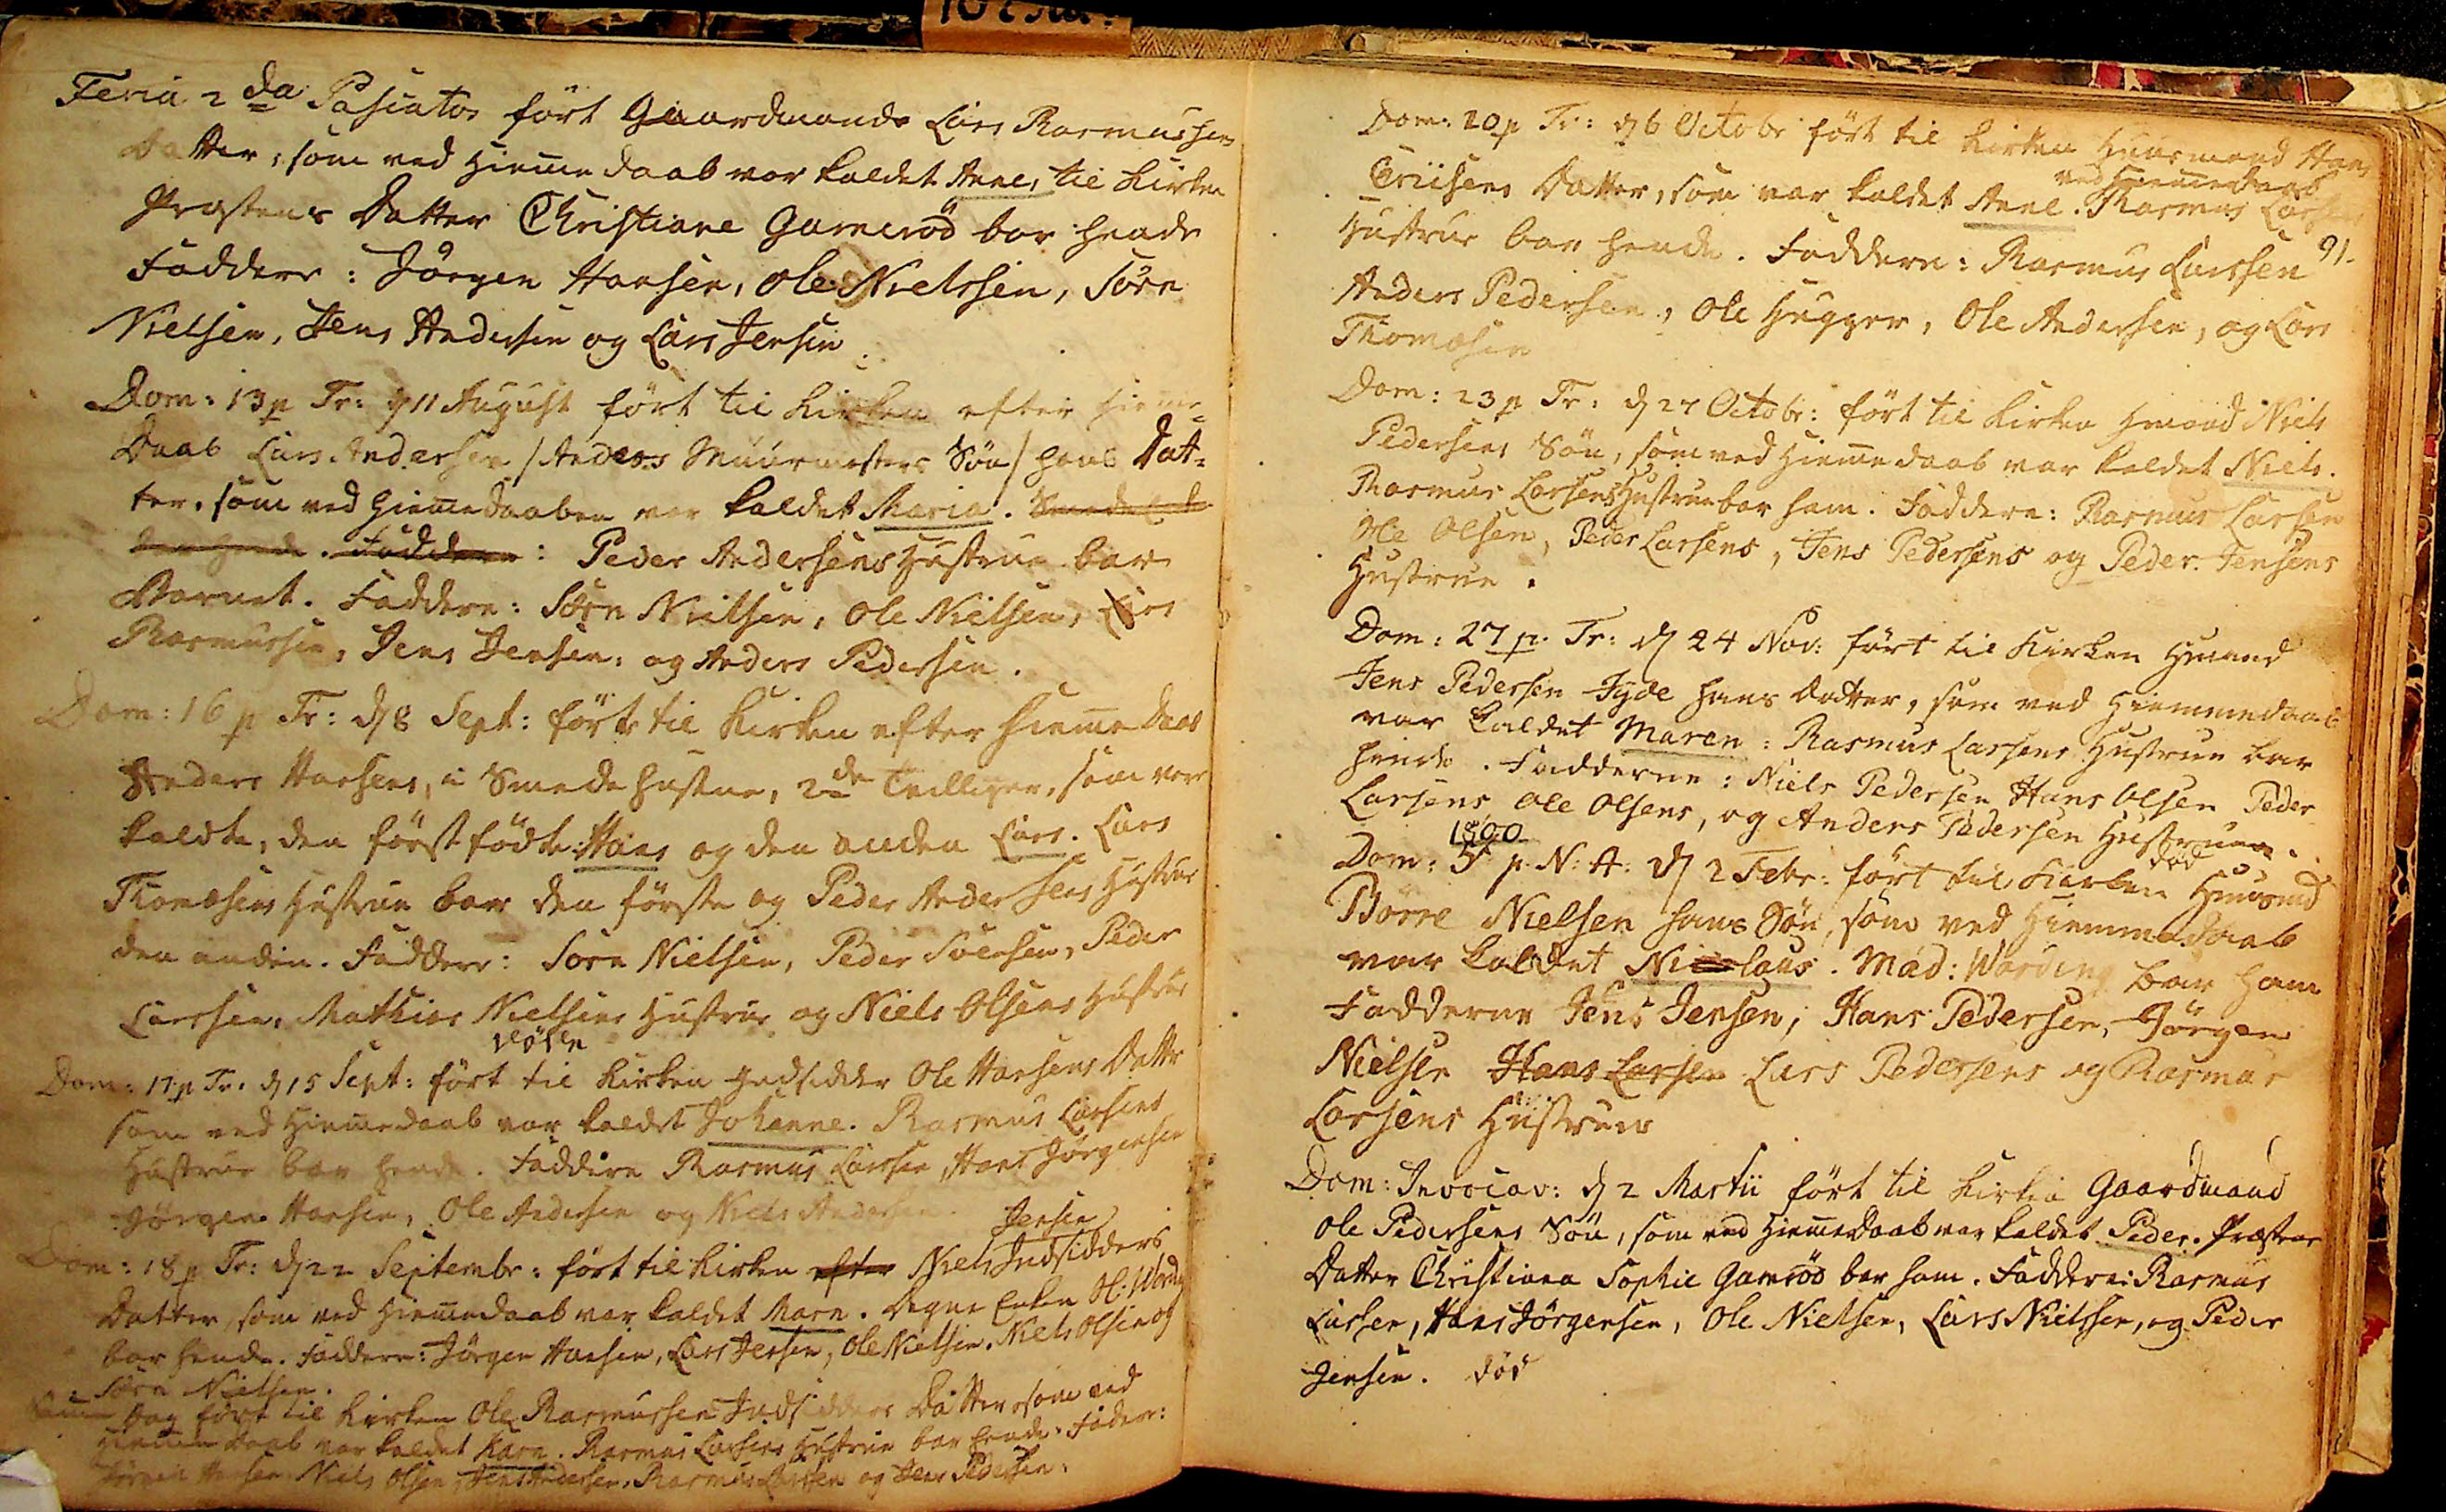Feria 2da Pascatus ført Gaardmand Lars Rasmussens
Datter, som ved Hiemmedaab var kaldet Anne, til Kirken
Præstens Datter Christiane Gamerød bar hende
Faddere: Jørgen Hansen, Ole Nielssen, Sørn
Nielsen, Jens Andersen og Lars Jensen.
Dom: 13 p Tr: d 11 August ført til Kirken efter Hiemme¬
Daab Lars Andersen / Anders Muurmesters Søn / hans Dat¬
ter, som ved Hiemmedaaben var kaldet Maria. Smede Enken
bar hende. Faddere: Peder Andersens Hustrue bar
Barnet. Faddere: Sørn Nielsen. Ole Nielsen, Lars
Rasmussen, Jens Jensen, og Anders Pedersen.
Dom: 16 p Tr: d 8 Sept: ført til Kirken efter Hiemme Daab
Anders Hansens, i Smedehusene, 2de Tvillinger, som vare
kaldte, den først fødte Hans og den anden Lars. Lars
Thomæsens Hustrue bar den første og Peder Andersens Hustrue
den anden. Faddere: Sørn Nielsen, Peder Svensen, Peder
Larssen, Mathias Nielsens Hustrue og Niels Olsens Hustrue
døde
Dom: 17 p Tr: d 15 Sept: ført til Kirken Indsidder Ole Hansens Datter
som ved Hiemmedaab var kaldet Johanne. Rasmus Larsens
Hustrue bar hende. Faddere Rasmus Larssen, Hans Jørgensen
Jørgen Hansen, Ole Andersen og Niels Andersen.
Jensen
Dom: 18 p Tr: d 22 Septembr: ført til Kirken efter Niels Indsidders
Datter, som ved Hiemmedaab var kaldet Marn. Degne Enken H: Wording
bar hende. Faddere. Jørgen Hansen, Lars Jensen, Ole Nielsen. Niels Olsen og
Sørn Nielsen.
Samme Dag ført til Kirken Ole Rasmussen Indsidders Datter som ved
Hiemme Daab var kaldet Karn. Rasmus Larsens Hustrue bar hende. Fadere:
Jørgen Hansen, Niels Olsen, Jens Andersen, Rasmus Larssen og Jens Pedersen.
91.
Dom: 20 p Tr: d 6 Octobr ført til Kirken Huusmand Hans
ved Hiemmedaab
Ericsens Datter, som var kaldet Anne. Rasmus Larssens
Hustrue bar hende. Faddere: Rasmus Larssen
Anders Pedersen, Ole Hugger, Ole Andersen, og Lars
Thomæsen.
Dom: 23 p Tr: d 27 Octobr: ført til Kirken Hmand Niels
Pedersens Søn, som ved Hiemmedaab var kaldet Niels.
Rasmus Larsens Hustrue bar ham. Faddere: Rasmus Larsen
Ole Olsen, Peder Larsens, Jens Pedersens og Peder Jensens
Hustrue.
Dom: 27 p. Tr: d 24 Nov: ført til Kirken Hmand
Jens Pedersen Jyde hans Datter, som ved Hiemmedaab
var kaldet Maren: Rasmus Larsens Hustrue bar
hende. Fadderne: Niels Pedersen Hans Olsen Peder
Larsens Ole Olsens, og Anders Pedersen Hustruer.
død
1800 
Dom: 5t p: N: A: d 2 Febr: ført til Kirken Huusmd
Børre Nielsen hans Søn, som ved Hiemmedaab
var kaldet Nicolaus. Mad: Wording bar ham
Fadderne Jens Jensen, Hans Pedersen, Jørgen
Nielsen Hans Larsen Lars Pedersens og Rasmus
Larsens Hustruer
Dom: Invocav: d 2 Martii ført til Kirken Gaardmand
Ole Pedersens Søn, som ved HiemmeDaab var kaldt Peder. Præstens
Datter Christiana Sophie Gamrød bar ham. Faddere: Rasmus
Larsen, Hans Jørgensen, Ole Nielsen, Lars Nielssen, og Peder
Jensen.  død
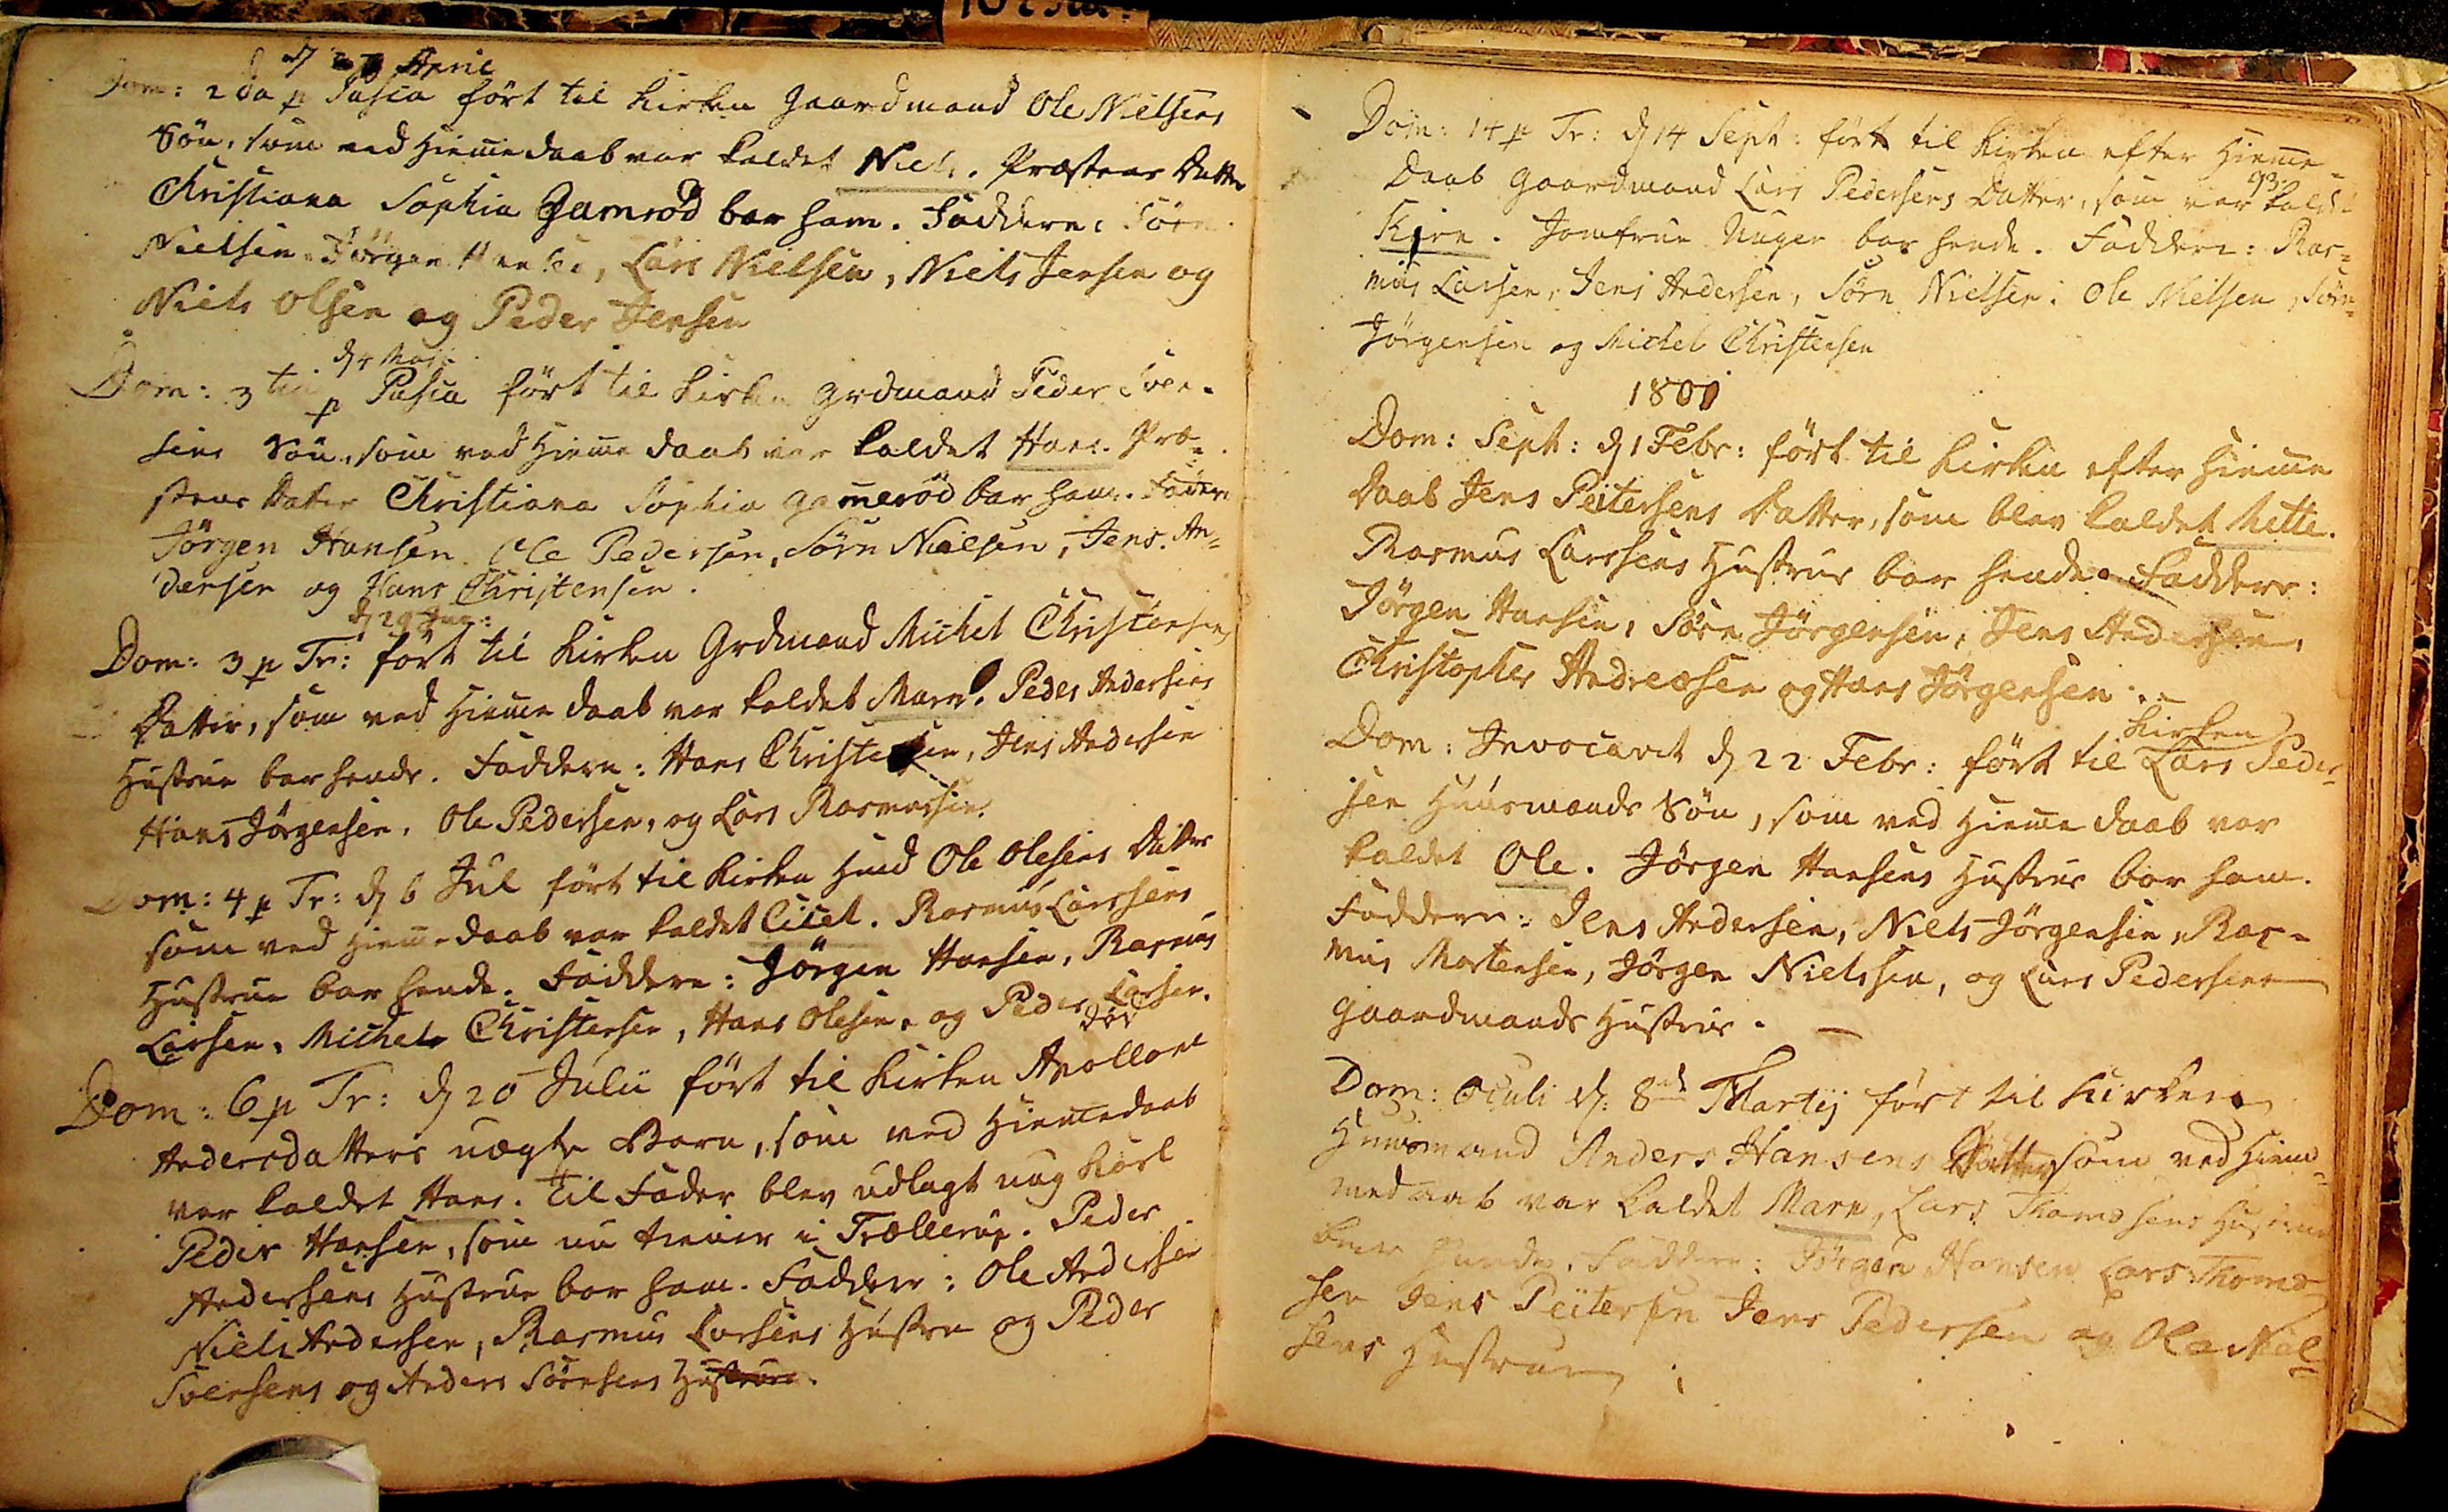d. 27 April
Dom: 2da p Pasca ført til Kirken Gaardmand Ole Nielsens
Søn, som ved Hiemmedaab var kaldet Niels. Præstens Datter
Christiana Sophia Gamrod bar ham. Fadderne Sørn
Nielsen, Jørgen Hansen, Lars Nielsen, Niels Jensen og
Niels Olsen og Peder Jensen
d 4 Maii
Dom: 3tia p Pasca ført til Kirken Grdmand Peder Sven¬
sens Søn, som ved Hiemme daab var kaldet Hans. Præ¬
stens Datter Christiana Sophia Gammerød bar ham. Fadere:
Jørgen Hansen. Ole Pedersen, Sørn Nielsen, Jens An¬
dersen og Hans Christensen.
d 29 Jun:
Dom: 3 p Tr: ført til Kirken Grdmand Michel Christensens
Datter, som ved Hiemme daab var kaldet Marn. Peder Andersens
Hustrue bar hende. Faddere: Hans Christensen, Jens Andersen
Hans Jørgensen, Ole Pedersen, og Lars Rasmussen.
Dom: 4 p Tr: d 6 Jul ført til Kirken Hmd Ole Olesens Datter
som ved Hiemmedaab var kaldet Cicel. Rasmus Larssens
Hustrue bar hende. Faddere: Jørgen Hansen, Rasmus
Larsen, Michel Christensen, Hans Olesen, og Peder Larsen.
død
Dom: 6 p Tr: d 20 Julii ført til Kirken Apollone
Andersdatters uægte Barn, som ved Hiemmedaab
var kaldet Hans. Til Fader blev udlagt ung Karl
Peder Hansen, som nu tiener i Trællerup. Peder
Andersens Hustrue bar ham. Faddere: Ole Andersen
Niels Andersen, Rasmus Larsens Hustru og Peder
Svensens og Anders Sørnsens Hustruer.
93.
Dom: 14 p Tr: d 14 Sept: ført til Kirken efter Hiemme¬
Daab Gaardmand Lars Pedersens Datter, som var kaldt
Karn. Jomfrue Unger bar hende. Faddere: Ras¬
mus Larsen, Jens Andersen, Sørn Nielsen, Ole Nielsen, Sørn
Jørgensen og Michel Christensen
1801
Dom: Sept: d 1 Febr: ført til Kirken efter hiemme
Daab Jens Peitersens Datter, som blev kaldet Mette.
Rasmus Larssens Hustrue bar hende. Faddere:
Jørgen Hansen, Sørn Jørgensen, Jens Andersen,
Christopher Andreæsen og Hans Jørgensen.
Kirken
Dom: Invocavit d 22 Febr: ført til Lars Peder¬
sen Huusmands Søn, som ved Hiemme daab var
kaldet Ole. Jørgen Hansens Hustrue bar ham.
Faddere: Jens Andersen, Niels Jørgensen, Ras¬
mus Mortensen, Jørgen Nielssen, og Lars Pedersens
Gaardmands Hustrue.
Dom: Oculi d: 8de Martij ført til Kirken
Huusmand Anders Hansens Datter som ved Hiem¬
medaab var kaldet Marn, Lars Thomæsens Hustrue
bar hende. Faddere: Jørgen Hansen Lars Thomæ¬
sen Jens Peitersen Jens Pedersen og Ole Niel¬
sens Hustrue.